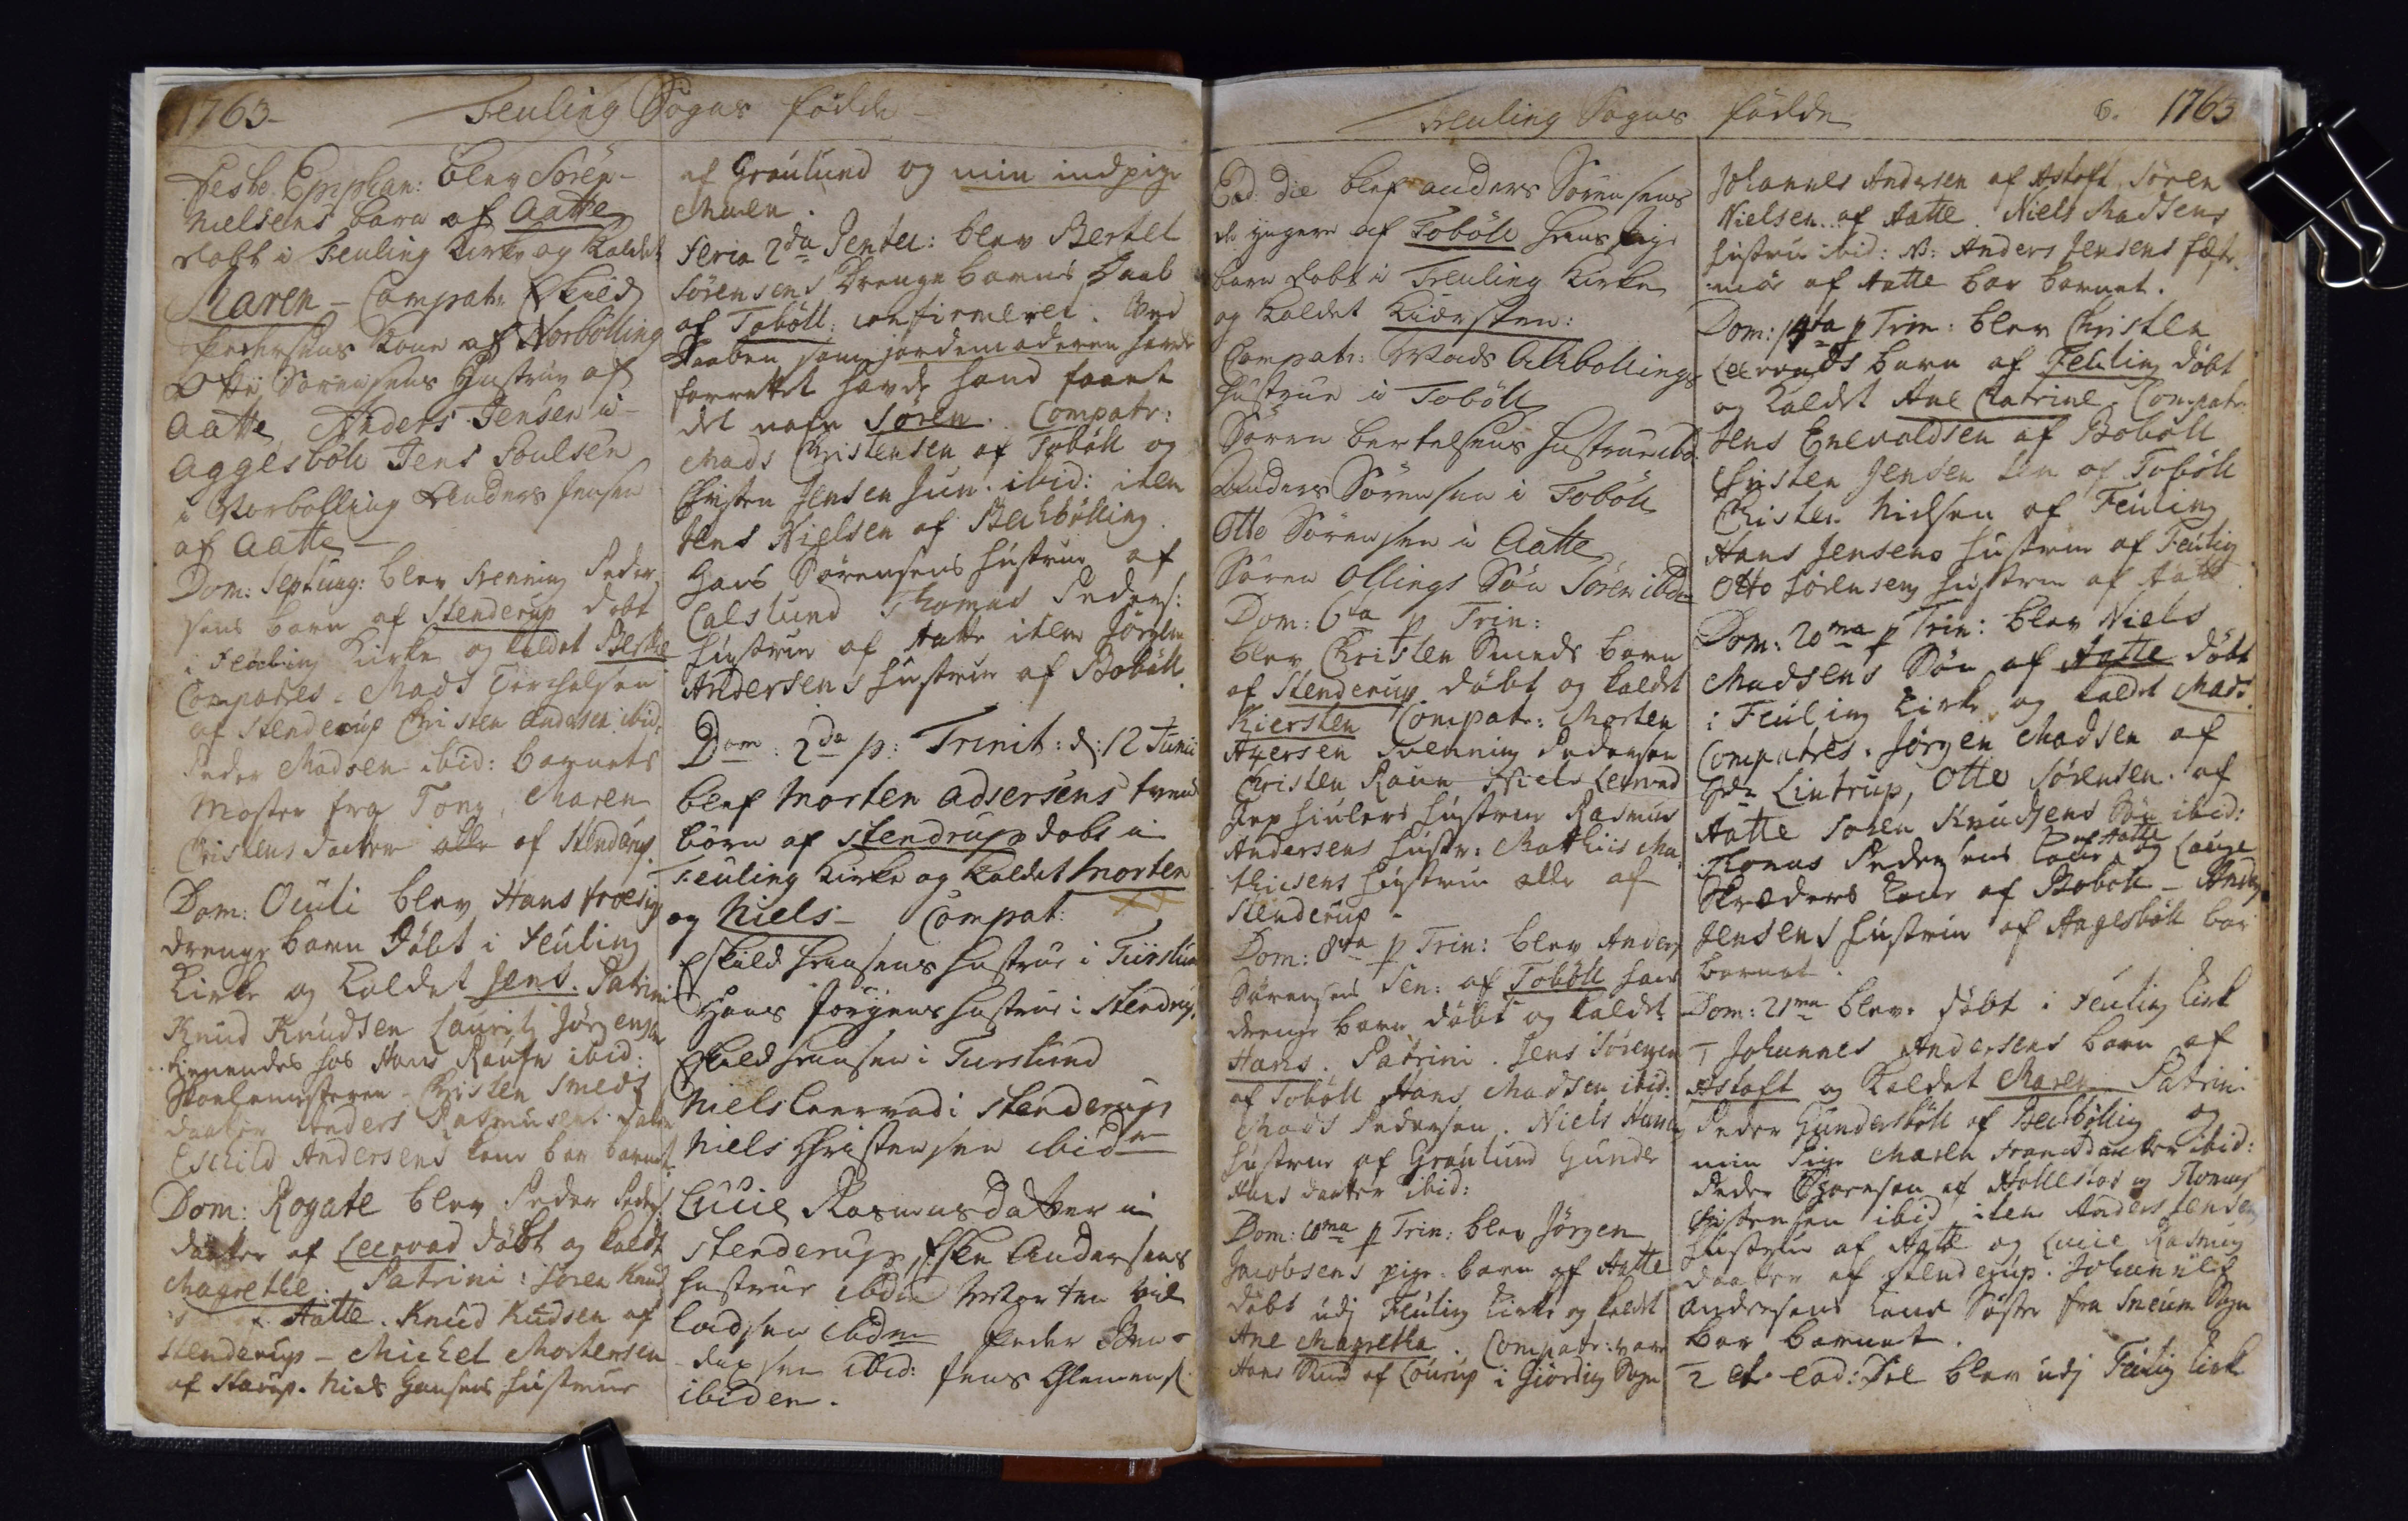1763-
Feuling Sogns fødde
Festo Epiphan: blev Søren
Nielsens barn af Aatte
døbt i Feuling Kirke og kaldet
Maren - Compat: Eskild
Pedersens Kone af Norbølling
Otte Sørensens Hustrue af
Aatte Anders Jensen i
Aggebøll Jens Poulsen
i Norbolling Anders Jensen
af Aatte
Dom: Septuag: blev Svenning Peder¬
sens Barn af Stenderup døbt
i Feuling Kirke og kaldet P##
Compatres: Mads Terchelsen
af Stenderup Christen Andersen ibid
Peder Madsen ibid: Barnets
Moster fra Tong, Maren
Christensdatter alle af Stenderup
Dom: Oculi blev Hans Frøsigs
Drenge barn døbt i Feuling
Kirke og kaldet Jens. Patrini
Knud Knudsen Lauritz Jørgensen
tienendes hos Hans Raufn ibid:
Skoelemesteren. Christen Smeds
Datter Anders Rasmusens Datter
Eschild Andersens Kone bar barnet
Dom: Rogate blev Peder Peders
Datter af Leervad døbt og kaldet
Magrethe. Patrini: Jøren Knuds
## af Aatte. Knud Kudsen af
Stenderup. Michel Mortensen
af Starup. Niels Hansens Hustrue
af Graulund og min indpige
Maren
Feria 2da Pentec: blev Bertel
Sørensens Drenge barns Daab
af Tobøl: confirmeret ved
Daaben som jordemoderen havde
forrettet havde hand faaet
det nafn Søren. Compatr:
Mads Christensen af Tobøl og
Christen Jensen Jun. ibid: item
Jens Nielsen af Bechbølling
Hans Sørensens Hustrue af
Calslund Thomas Peders:
Hustrue af Aatte item Jørgen
Andersens Hustrue af Bobøll
Dom: 2da p: Trinit: d: 12 Junii
blef Morten Adsersens tvende
børn af Stendrup døbt i
Feuling Kirke og kaldet Morten
og Niels Compat:
Eskild Hansens Hustrue i Tiirslund
Hans Jørgens Hustrue i Stendrup.
Eskild Hansen i Tiirslund
Niels Leervad i Stenderup
Niels Christensen ibid##
Lucie Rasmusdatter i
Stenderup, Eske Andersens
Hustrue ibid Morten Vil
ladsen ibid## Peder Ben¬
dixsen ibid: Jens Chlemens
ibiden.
Feuling Sogns føadde
Eod: Die blef Anders Sørensens
den yngere af Tobøl, Hans Pige
barn døbt i Feuling Kirke
og Kaldet Kiersten:
Compatr: Mads AAbøllings
Hustrue i Tobøll
Søren Bertelsens Hustrue ibid
Anders Sørensen i Tobøll
Otte Sørensen i Aatte
Søren Ollings Søn Søren ibidem
Dom: 6ta p: Trin:
blev Christen Smeds barn
af Stenderup. døbt og kaldet
Kiersten Compatr: Morten
Adsersen Svenning Pedersen
Christen Raun Niels Leervad
Jep Hiulers Hustrue Rasmus
Andersens Hustr: Mathiis Ma¬
thiesens Hustrue alle af
Stenderup
Dom: 8va p Trin: blev Anders
Sørensen Sen: af Tobøll hans
drenge barn døbt og kaldet
Hans. Patrini Jens Sørensen
af Tobøll Hans Madsen ibid:
Mads Pedersen. Niels Hansens
Hustrue af Graulund Gunder
Hans daatter ibid:
Dom: 10ma p Trin: blev Jørgen
Jacobsens pige barn af Aatte
døbt udi Feuling Kirke og kaldet
Ane Magretha Compatr: vare
Hans Smed af Lourup i Giording Sogn
6.
1763
Johannes Andersen af Astoft, Søren
Nielsen af Aatte. Niels Madsens
Hustru ibid: NB: Anders Jensens Fæste¬
mø af Aatte bar barnet.
Dom: 14ta p Trin: blev Christen
Leervads barn af Feuling døbt
og kaldet Ane Catrine. Compatr:
Jens Enevoldsen af Bobøll
Christen Jensen Sen og Tobøl
Chisten Nielsen af Feuling
Hans Jensens Hustrue af Feuling
Otto Sørensens Hustrue af Aatte
Dom: 20ma p Trin: blev Niels
Madsens Søn af Aatte døbt
i Feuling Kirke og kaldet Mads.
Compatres. Jørgen Madsen af
Sdr Lintrup, Otte Sørensen af
Aatte Søren Knudsens Søn ibid:
af Aatte
Thomas Pedersens Kone og Lauge
Skræders Kone af Bobøll - Anders
Jensens Hustrue af Aagesbæll bar
barnet.
Dom: 21ma blev døbt i Feuling Kirk
Johannes Andersens Barn af
Astoft og Kaldet Maren Patrini
Peder Gundersbøll af Bechbølling og
min Pige Maren Frandsdaatter ibid:
Peder ##nsen af Holleskov og Thomas
Christensen ibid item Anders Jensens
Hustrue af Aatte og Lucie Rasmus
Daatter af Stenderup, Johannes
Andersens Kone Søster fra Sneum Sogn
bar barnet.
2 ## eod: Die blev udi Feuiling Kirke
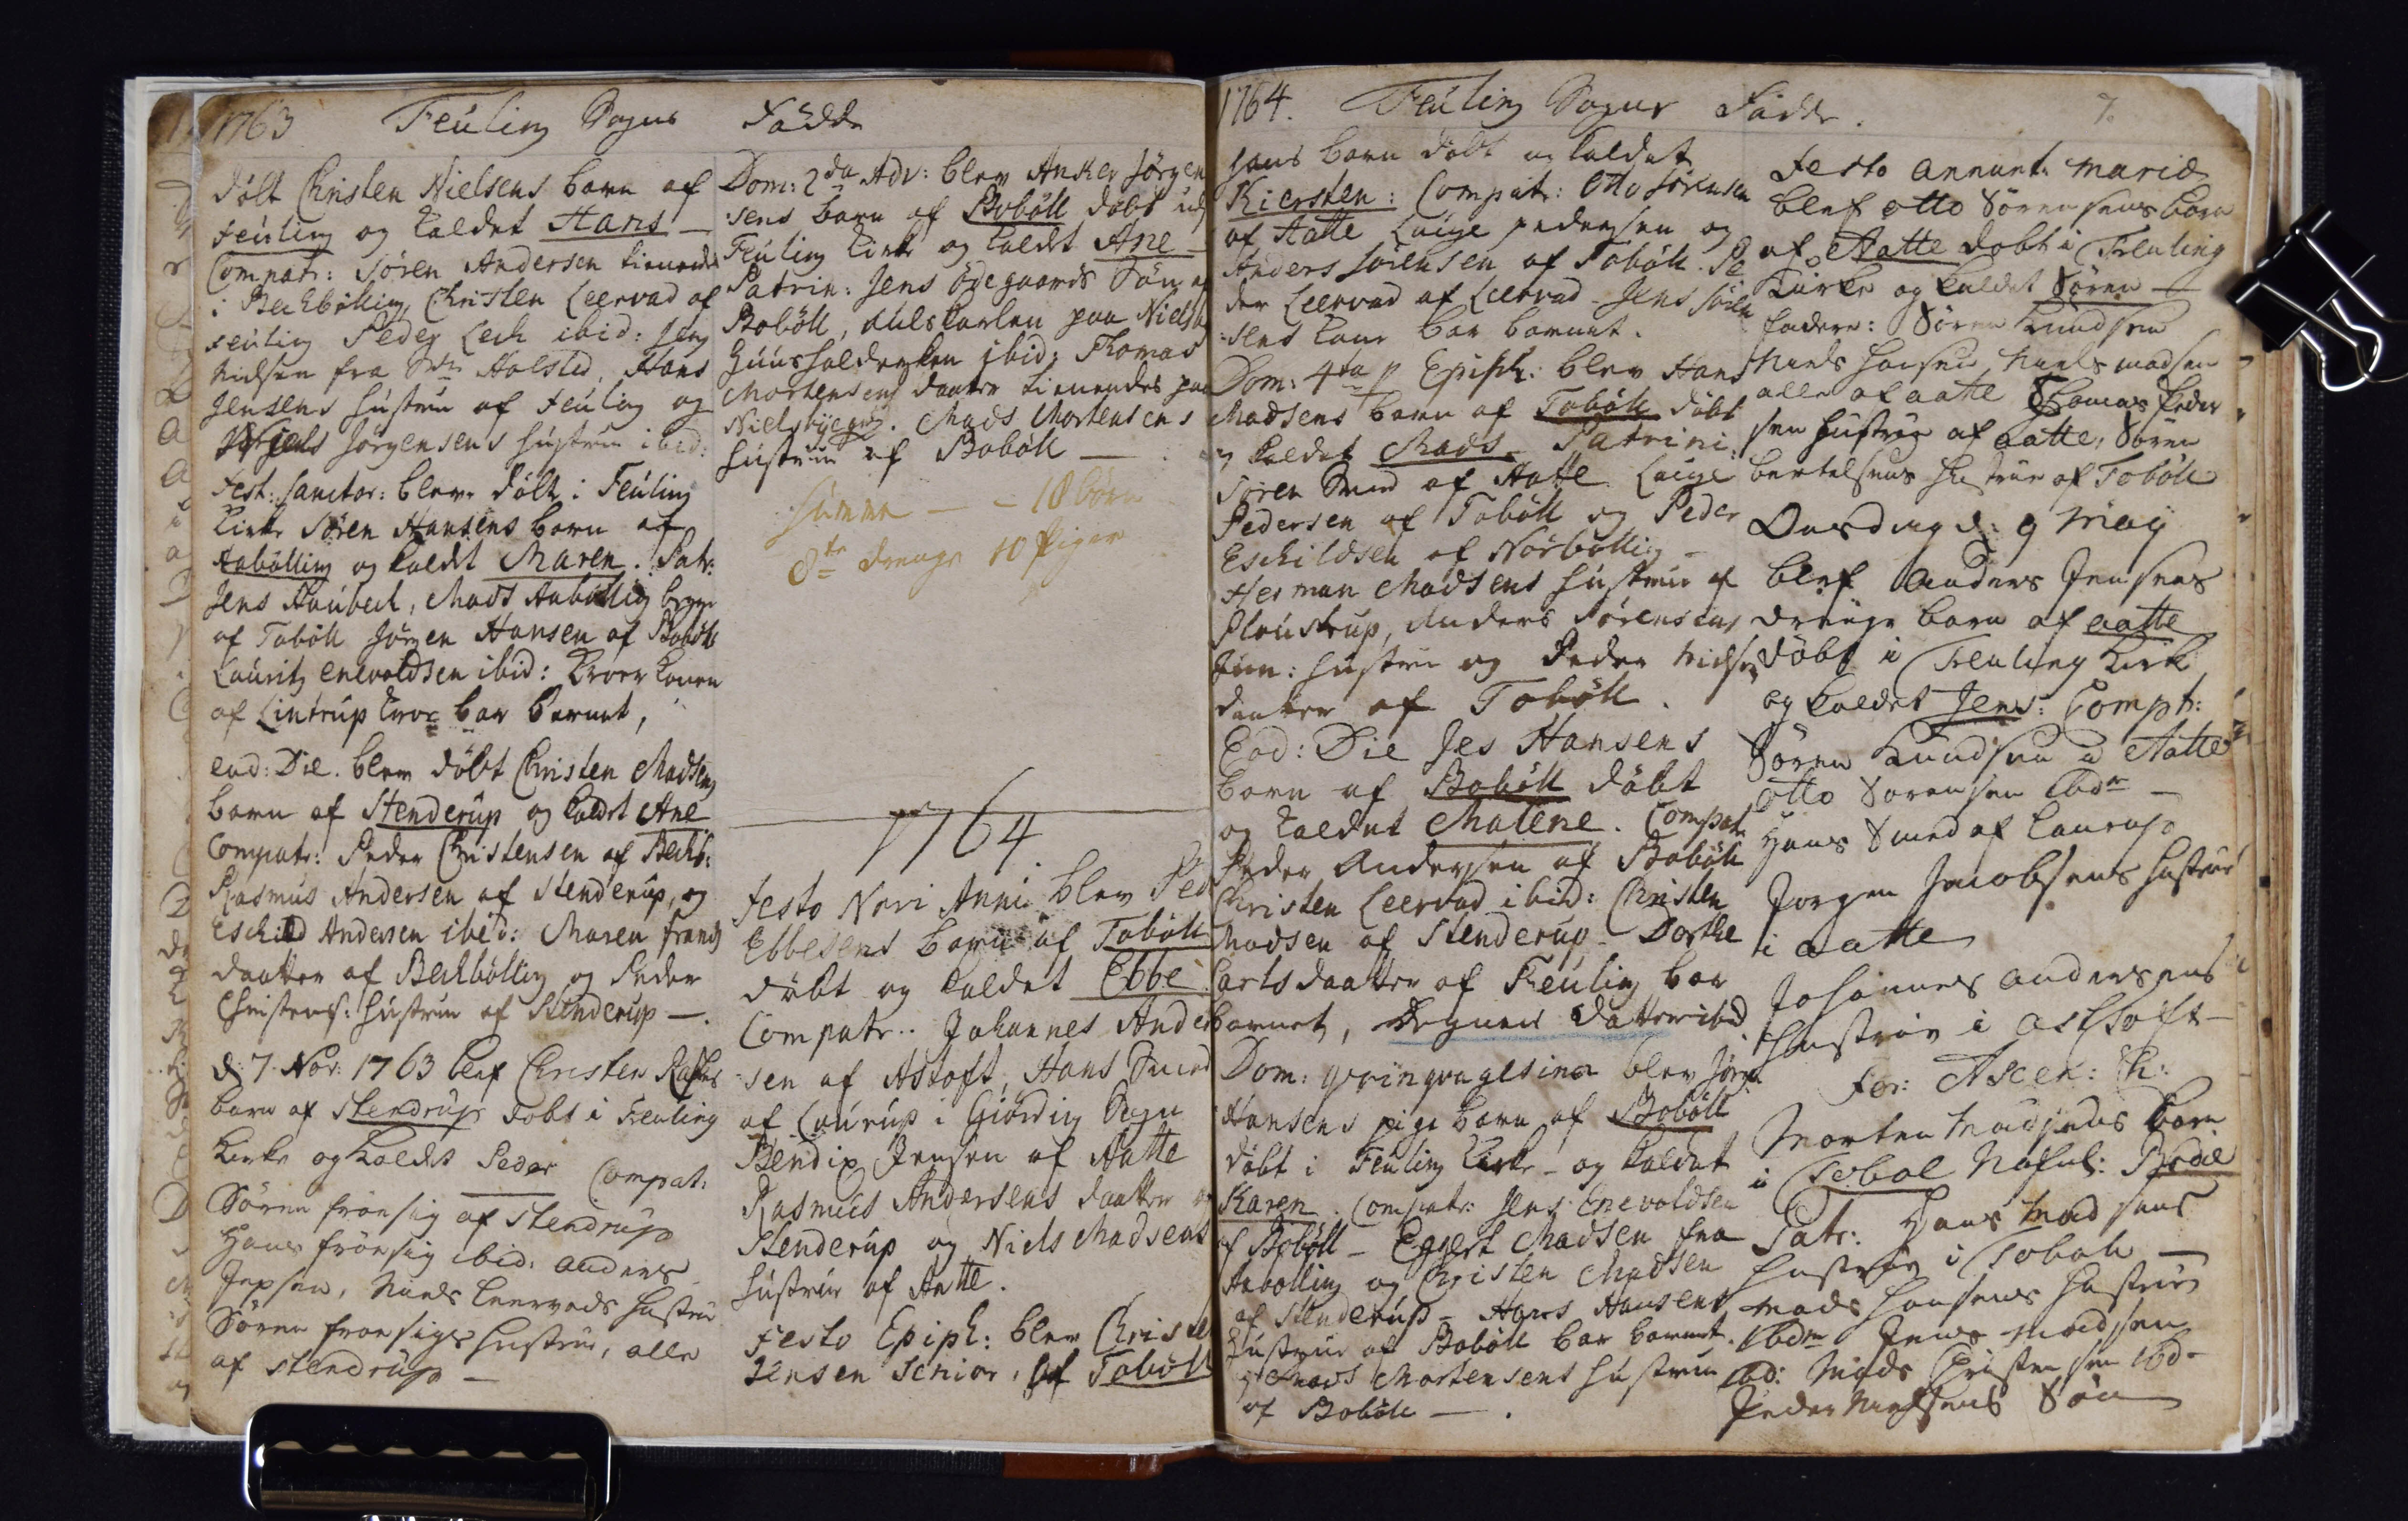Feuling Sogns
1763
døbt Christen Nielsens barn af
Feuling og kaldet Hans -
Compatr: Søren Andersen tienende
i Bechbølling, Christen Leervad af
Feuling Peder Lech ibid: Jens
Nielsen fra Sdr Holsted. Hans
Jensens Hustrue af Feuling og
Jørgen Jørgensens Hustrue ibid:
Fest. Sanctor bleve døbt i Feuling
Kirke Søren Hansens barn af
Aabølling og kaldet Maren. Patr:
Jens Raubech, Mads Aabølling begge
af Tobøll Jørgen Hansen af Bobøll
Lauritz Envoldsen ibid: Kroer Konen
af Lintrup Kroe bar barnet,
Eod: Die blev døbt Christen Madtsens
Barn af Stenderup og kaldet Ane
Compatr: Peder Christensen af Bechs:
Rasmus Andersen af Stenderup, og
Eschild Andersen ibid: Maren Frands
Daatter af Bechbølling og Peder
Christens: Hustrue af Stenderup
d: 7 Nov: 1763 blef Christen Rases
barn af Stendrup døbt i Feuling
Kirke og kaldet Peder Compat:
Søren Frøesig af Stendrup
Hans frøesig ibid: Anders
Jepsen, Mads Leervads Hustrue
Søren Frøesigs Hustrue, alle
af Stendrup
Faødde
Dom: 2da Adv: blev Anker Jørgen
sens Barn af Bobøll døbt udi
Feuling Kirke og kaldet Ane
Patrin: Jens ##degaards Søn af
Bobøll, aulskarlen paa Nielby
Huusholdersen ibid; Thomas
Mortensens Daatter tienendis paa
Nielsbyegrd. Mads Mortensens
Hustrue af Bobøll.
Summa - - 18 Børn
8te drenge 10 Piger
1764
Festo Novi Anni blev Ped
Ebbesens Barn af Tobøll
døbt og kaldet Ebbe
Compatr: Johannes Ander
sen af Astoft. Hans Smed
af Lourup i Giørding Sogn
Bendix Jensen af Aatte
Rasmus Andersens Daatter af
Stenderup og Niels Madsens
Hustrue af Aatte
Festo Epiph: blev Christen
Jensen Senior af Tobøll
1764.
Feuling Sogns Fødde
hans Barn døbt og kaldet
Kiersten: Compat: Otto Sørensen
af Aatte Lauge Pedersen og
Anders Sørensen af Tobøll. Pe¬
der Leervad af Leervad Jens Søren¬
sens Kone bar barnet.
Dom 4ta p Epiph: blev Hans
Madsens Barn af Tobøll døbt
og kaldet Mads Patrini:
Søren Smed af Aatte Lauge
Pedersen af Tobøll og Peder
Eschildsen af Nørbølling
Herman Madsens Hustrue i
Ploustrup, Anders Sørensens
Jun: Hustrue og Peder Nielsens
Daatter af Tobøll
Eod: Die Jes Hansens
Barn af Bobøll døbt
og kaldet Malene. Compatr
Peder Andersen af Bobøll
Christen Leervad ibid: Christen
Madsen af Stenderup, Dorthe
Carlsdaatter af Feuling bar
Barnet, Degnens Datter ibd
Dom: Qvinqvagesima blev Jørgen
Hansens pige Barn af Bobøll
døbt i Feuling Kirke - og kaldet
Karen. Compatr: Jens Enevoldsen
af Bobøll - Eggert Madsen fra
Aabølling og Christen Madsen
af Stenderup - Hans Hansens
Hustrue af Bobøll bar barnet.
og Mads Mortensens Hustrue
af Bobøll
7.
Festo Annunt: Mariæ
blef Otte Sørensens barn
af Aatte døbt i Feuling
Kirke og kaldet Søren -
Faddere: Søren Knudsen
Niels Hansen, Niels Madsen
alle af aatte Thomas Peder
sen Hustrue af aatte, Søren
Bertelsens Hustrue af Tobøll
Onsdag d: 9 Maij.
blef Anders Jensens
Drenge barn af aatte
døbt i FGeuling Kirk
og kaldet Jens: Compt:
Søren Knudsen i Aatte
Otto Sørensen ibid##
Hans Smed af Laurup
Jørgen Jacobsens Hustrue
i aatte
Johannes Andersens
Hustroe i Astoft
Fer: Ascen: Chr:
Morten Madsens Barn
i Tobol Nafnl: Bodil
Patr:: Hans Madsens
Hustrue i Toboll
Mads Hansens Hustrue
ibdm Jens Madsen
ibd: Mads Christensen ibd
Peder Madsens Søn